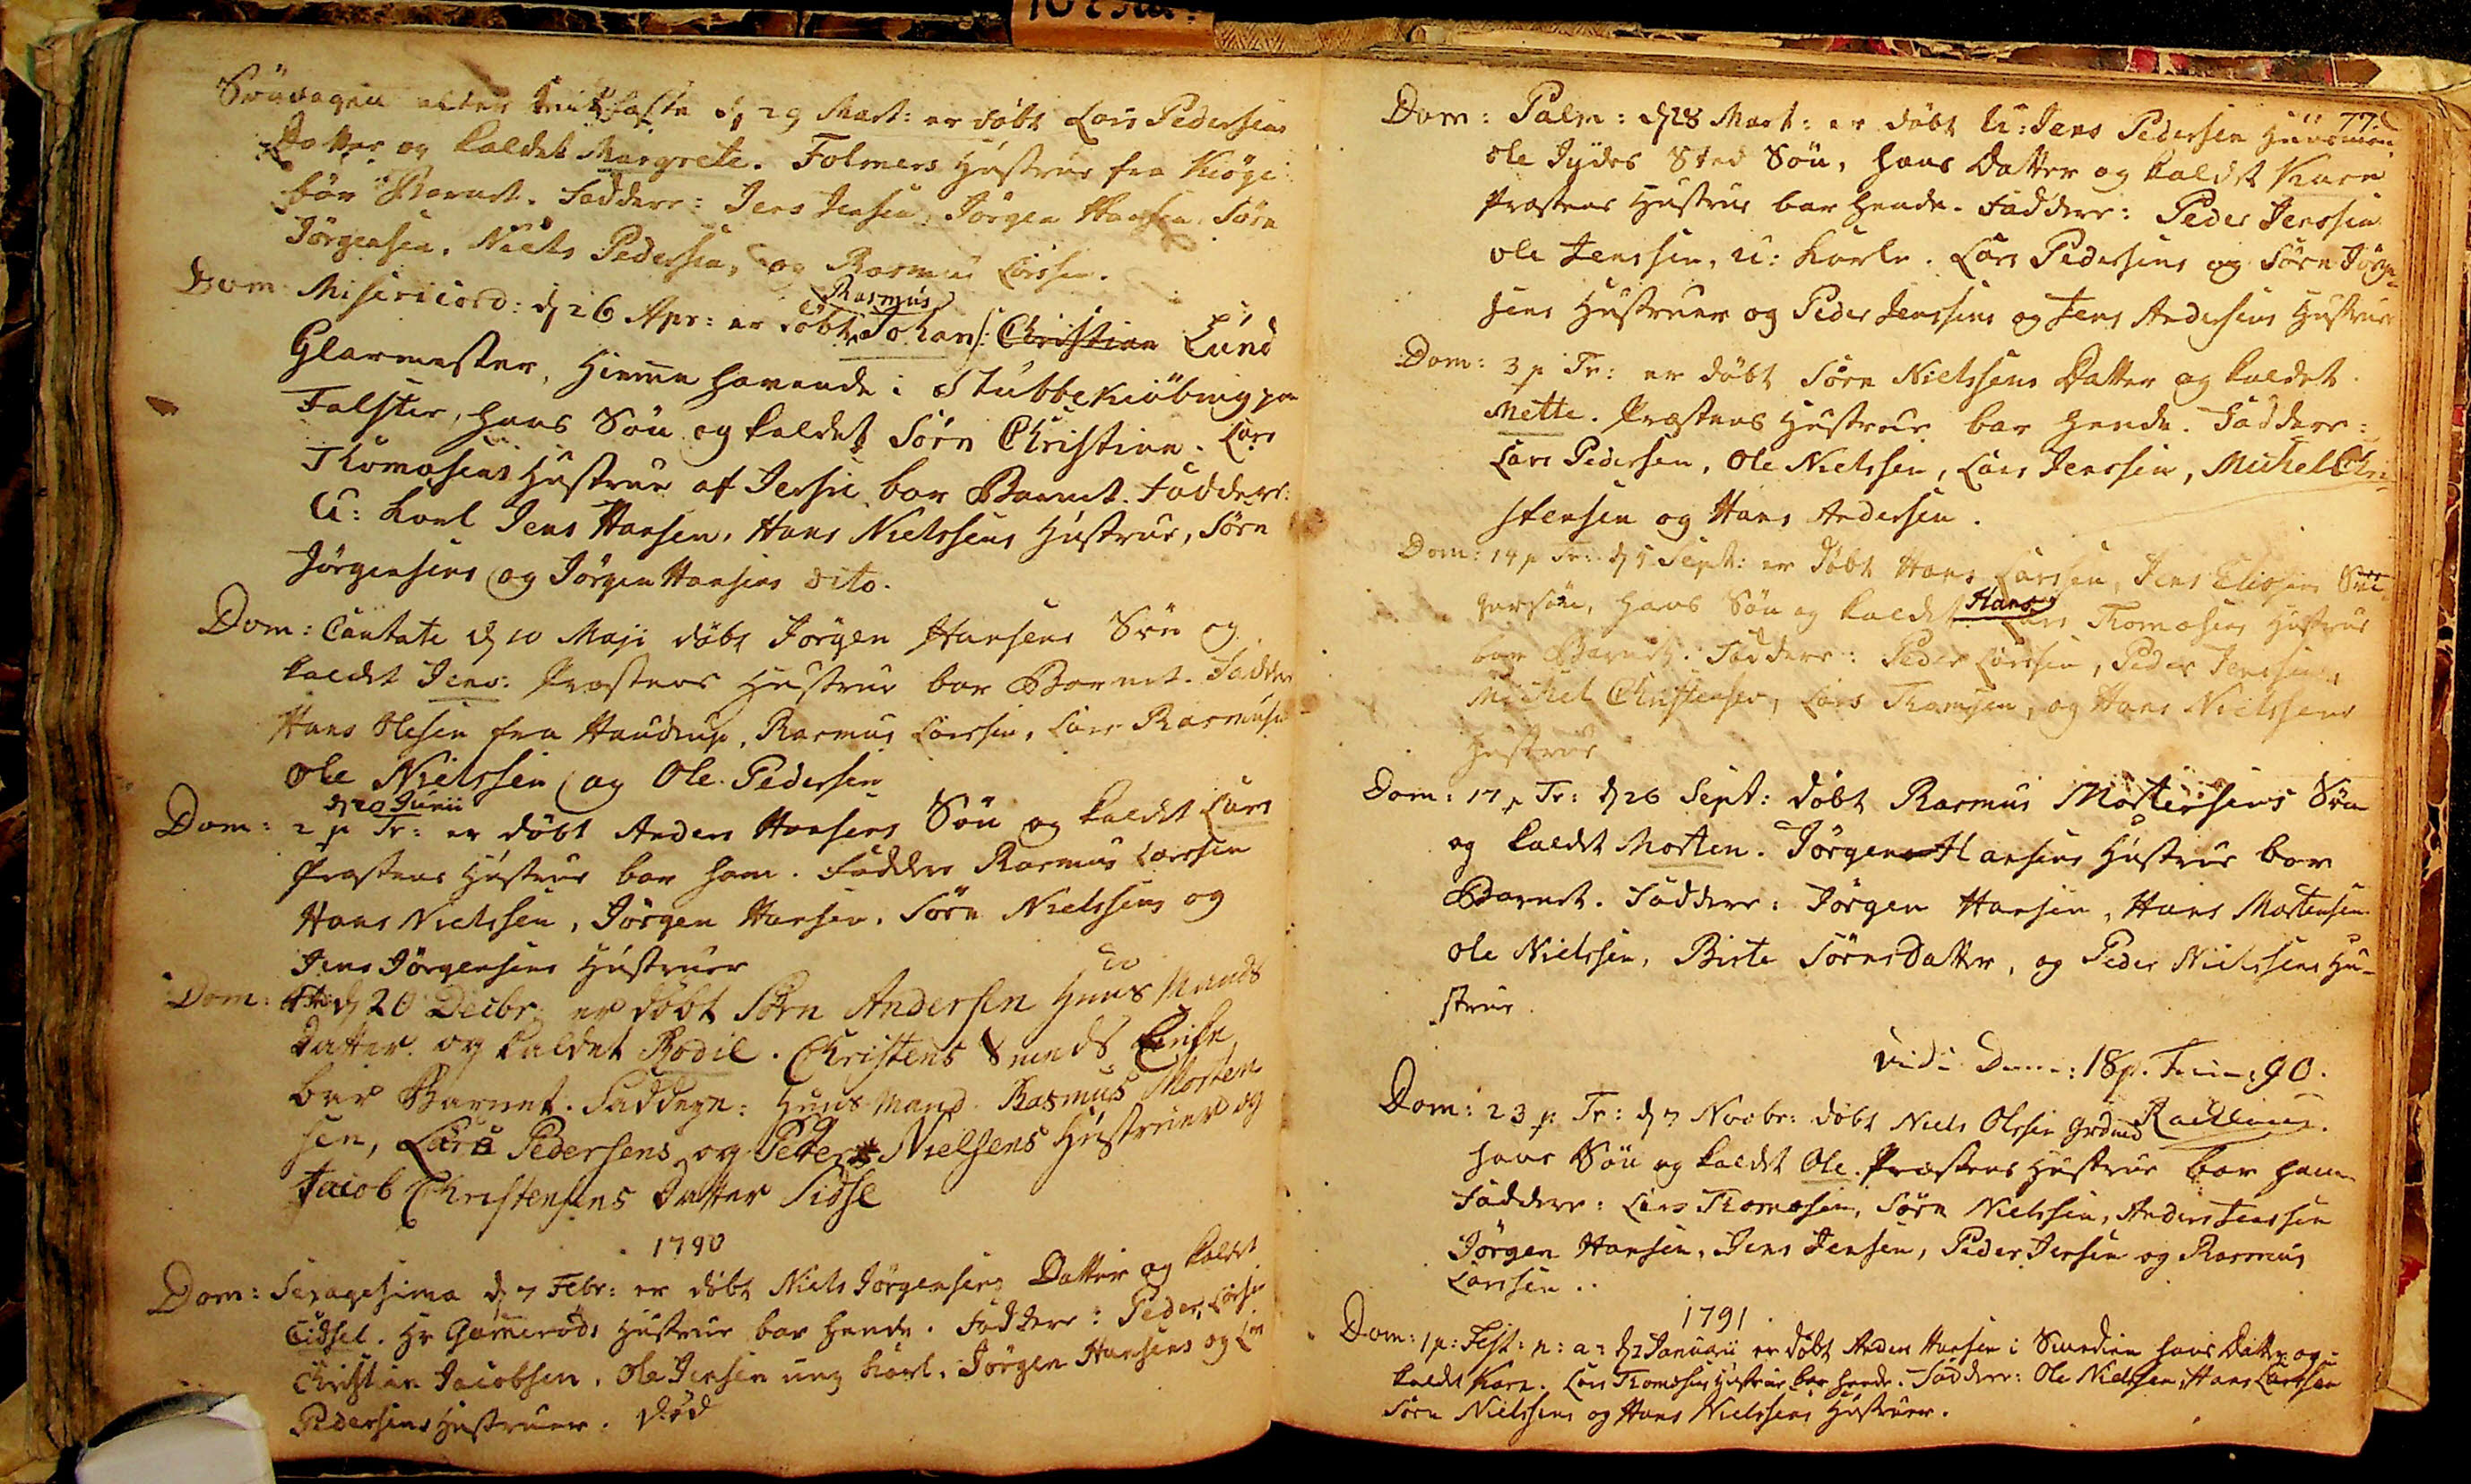Søndagen efter Midfaste d 29 Mart: er døbt Lars Pedersens
Datter og kaldet Margrete. Folmers Hustrue fra Kiøge
bar Barnet. Faddere: Jens Jensen, Jørgen Hansen, Sørn
Jørgensen, Niels Pedersen, og Rasmus Larssen.
Rasmus 
Dom: Misericord: d 26 Apr: er døbt Johans: Christian Lund
Glarmester, hiemmehavende i StubbeKiøbing paa
Falster, hans Søn og kaldet Sørn Christien. Lars
Thomæsens Hustrue af Jersie bar Barnet. Faddere:
U: Karl Jens Hansen, Hans Nielssens Hustrue, Sørn
Jørgensens og Jørgen Hansens dito
Dom: Cantate d 10 Maji døbt Jørgen Hansens Søn og
kaldet Jens: Præstens Hustrue bar Barnet. Faddere
Hans Olesen fra Haudrup, Rasmus Larssen, Lars Rasmussen
Ole Nielssen og Ole Pedersen
d 20 Junii
Dom: 2 p Tr: er døbt Anders Hansens Søn og kaldt Lars
Præstens Hustrue bar ham. Faddere Rasmus Larssen
Hans Nielssen, Jørgen Hansen, Sørn Nielssens og
Jens Jørgensens Hustruer
Dom: 4 Ad: d 20 Decbr: er døbt Sørn Andersen Huus Mands
Datter og kaldet Bodil. Christens Smeds Enke
bar Barnet. Faddere: Huus-Mand Rasmus Morten
sen, Lars Pedersens og Peder Nielsens Hustruer og
Jacob Christensens Datter Sidse
1790
Dom: Sexagesima d 7 Febr: er døbt Niels Jørgensens Datter og kaldet
Cidsel. Hr Gammerøds Hustrue bar hende. Faddere: Peder Larsen
Christian Jacobsen, Ole Jensen ung karl, Jørgen Hansens og Lars
Pedersens Hustruer. død.
77.
Dom: Palm: d 28 Mart: er døbt U: Jens Pedersen Huusmand
Ole Jydes Sted Søn, Hans Datter og kaldet Karn.
Præstens Hustrue bar hende. Faddere: Peder Jenssen
Ole Jenssen, u:Karle. Lars Pedersens og Sørn Jørgn¬
sens Hustruer og Peder Jenssens og Jens Andersens Hustruer
Dom: 3 p Tr: er døbt Sørn Nielssens Datter og kaldet 
Mette. Præstens Hustrue bar hende. Faddere:
Lars Pedersen, Ole Nielssen, Lars Jenssen, Michel Chri¬
stensen og Hans Andersen.
Dom: 14 p Tr: d 5 Sept: er døbt Hans Larssen, Jens Eliæsens Svi¬
Hans
gersøn, hans Søn og kaldet. Lars Thomæsens Hustrue
bar Barnet. Faddere: Peder Larssen, Peder Jenssen,
Michel Christensen, Lars Thomsen, og Hans Nielssens
Hustrue.
Dom: 17 p Tr: d 26 Sept: døbt Rasmus Mortensens Søn
og kaldet Morten. Jørgen Hansens Hustrue bar
Barnet. Faddere: Jørgen Hansen, Hans Mortensen,
Ole Nielssen, Birte Sørnsdatter, og Peder Nielssens Hu¬
strue.
Vidi Dom: 18 p. Trin: 90.
Rachling
Dom: 23 p Tr: d 7 Novbr: døbt Niels Olssen Grdmd
hans Søn og kaldt Ole. Præstens Hustrue bar ham
Faddere: Lars Thomæsen, Sørn Nielssen, Anders Jenssen
Jørgen Hansen, Jens Jensen, Peder Jensen og Rasmus
Larssen.
1791.
Dom: 1 p: Fest: n: a: d 2 Januarii er døbt Anders Hansen i Smedien hans Datter og
kaldet Karn. Lars Thomæsens Hustrue bar hende. Faddere: Ole Nielssen, Hans Larssen
Sørn Nielssens og Hans Nielssens Hustruer.
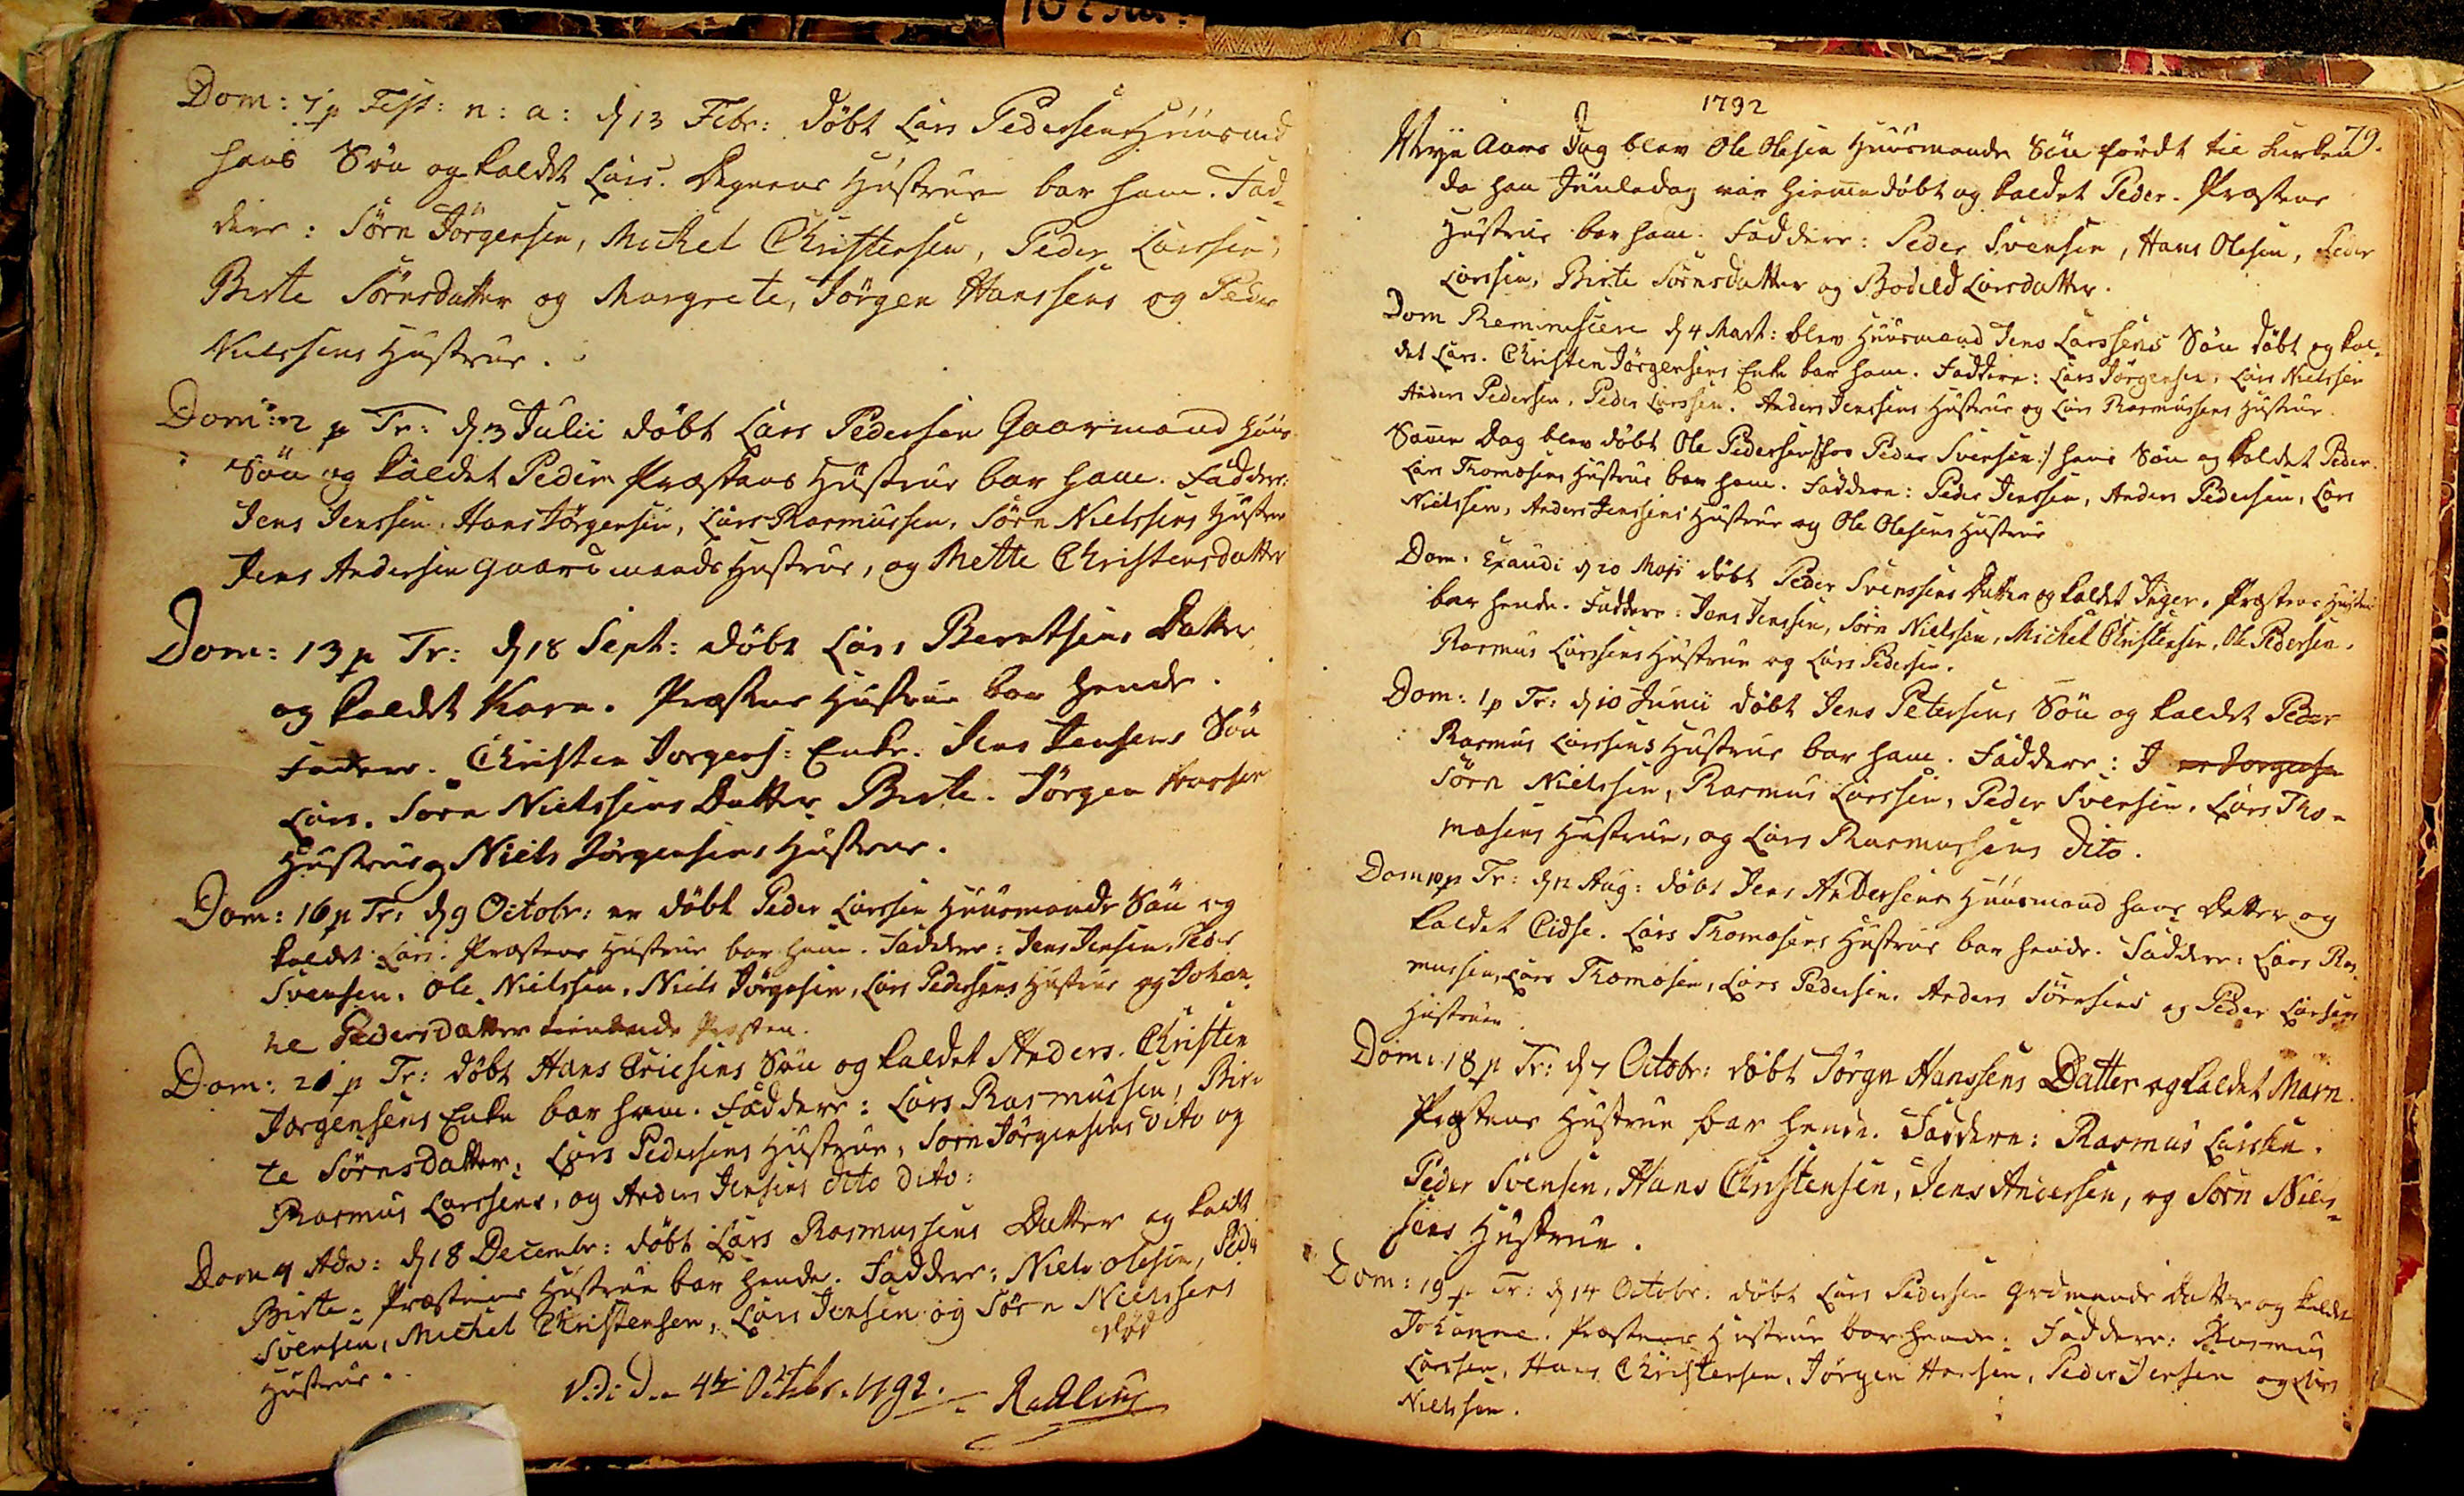Dom: 7 p Fest: n: a: d 13 Febr: døbt Lars Pedersen Huusmd
hans Søn og kaldt Lars. Degnens Hustrue bar ham. Fad¬
dere: Sørn Jørgensen, Michel Christensen, Peder Larssen,
Birte Sørnsdatter og Margrete, Jørgen Hanssens og Peder
Nielssens Hustrue.
Dom: 2 p Tr: d 3 Julii døbt Lars Pedersen Gaardmand hans
Søn og kaldet Peder. Præstens Hustrue bar ham. Faddere:
Jens Jenssen, Hans Jørgensen, Lars Rasmussen, Sørn Nielssens Hustrue
Jens Andersen Gaardmands Hustrue, og Mette Christensdatter
Dom: 13 p Tr: d 18 Sept: døbt Lars Berntsens Datter
og kaldet Karn. Præstens Hustrue bar hende.
Fadere: Christen Jørgens: Enke. Jens Jensens Søn
Lars. Søren Nielssens Datter Birte. Jørgen Hansen
Hustrue og Niels Jørgensens Hustrue.
Dom: 16 p Tr: d 9 Octobr: er døbt Peder Larssen Huusmands Søn og
kaldt Lars. Præstens Hustrue bar ham. Faddere: Jens Jensen, Peder
Svensen, Ole Nielssen, Niels Jørgnsen, Lars Pedersens Hustrue og Johan¬
ne Pedersdatter tienende Præsten.
Dom: 21 p Tr: døbt Hans Ericsens Søn og kaldet Anders. Christen
Jørgensens Enke bar ham. Faddere: Lars Rasmusen, Bir¬
te Sørnsdatter, Lars Pedersens hustrue, Sørn Jørgensens dito og
Rasmus Larssens, og Anders Jensens dito dito:
Dom 4 Adv: d 18 Decembr: døbt Lars Rasmussens Datter og kaldt
Birte. Præstens Hustrue bar hende. Faddere: Niels Olesen, Peder
Svensen, Michel Christensen, Lars Jensen og Sørn Nielssens
Hustrue.        
død
Vidi den 4te Octobr: 1792 ./.
Rachling
79.
1792
Nye Aars Dag blev Ole Olesen Huusmands Søn førdt til Kirken
da han Juuledag var hiemmedøbt og kaldet Peder. Præstens
Hustrue bar ham. Faddere: Peder Svensen, Hans Olesen, Peder
Larssen, Birte Sørnsdatter og Bodild Larsdatter.
Dom Reminiscere d 4 Mart: blev Huusmand Jens Larssens søn døbt og kal
det Lars. Christen Jørgensens enke bar ham. Faddere: Lars Jørgensen, Lars Nielssen
Anders Pedersen. Peder Larssen. Anders Jenssens Hustrue og Lars Rasmussens Hustrue.
Samme Dag blev døbt Ole Pedersens /: hos Peder Svensen :/ hans Søn og kaldet Peder.
Lars Thomæsens Hustrue bar ham. Faddere: Peder Jenssen, Anders Pedersen, Lars
Nielssen, Anders Jenssens Hustrue og Ole Olesens Hustrue
Dom: Exaudi d 20 Maji døbt Peder Svenssens Datter og kaldet Inger. Præstens Hustrue
bar hende. Faddere: Jens Jenssen, Sørn Nielssen, Michel Christensen, Ole Pedersen,
Rasmus Larssens Hustrue og Lars Pedersen.
Dom: 1 p Tr: d 10 Junii døbt Jens Petersens Søn og kaldet Peder
Rasmus Larssens Hustrue bar ham. faddere: Jens Jørgensen
Sørn Nielssen, Rasmus Larssen, Peder Svensen, Lars Tho¬
mæsens Hustrue, og Lars Rasmussens dito.
Dom: 10 p Tr: d 12 Aug: døbt Jens Andersens Huusmand hans Datter og
kaldet Cidse. Lars Thomæsens Hustrue bar hende. Faddere: Lars Ras
mussen, Lars Thomæsen, Lars Pedersen, Anders Sørnsens og Peder Larsens
Hustruer.
Dom: 18 p Tr: d 7 Octobr: døbt Jørgn Hanssens Datter og kaldet Marn.
Præstens Hustrue bar hende. Faddere: Rasmus Larssen,
Peder Svensen, Hans Christensen, Jens Andersen, og Sørn Niel¬
sens Hustrue.
Dom: 19 p Tr: d 14 Octobr: døbt Lars Pedersen Grdmands Datter og kaldet
Johanne. Præstens Hustrue bar hende. Faddere: Rasmus
Larssen, Hans Christensen, Jørgen Hansen, Peder Jensen og Lars
Nielssen.